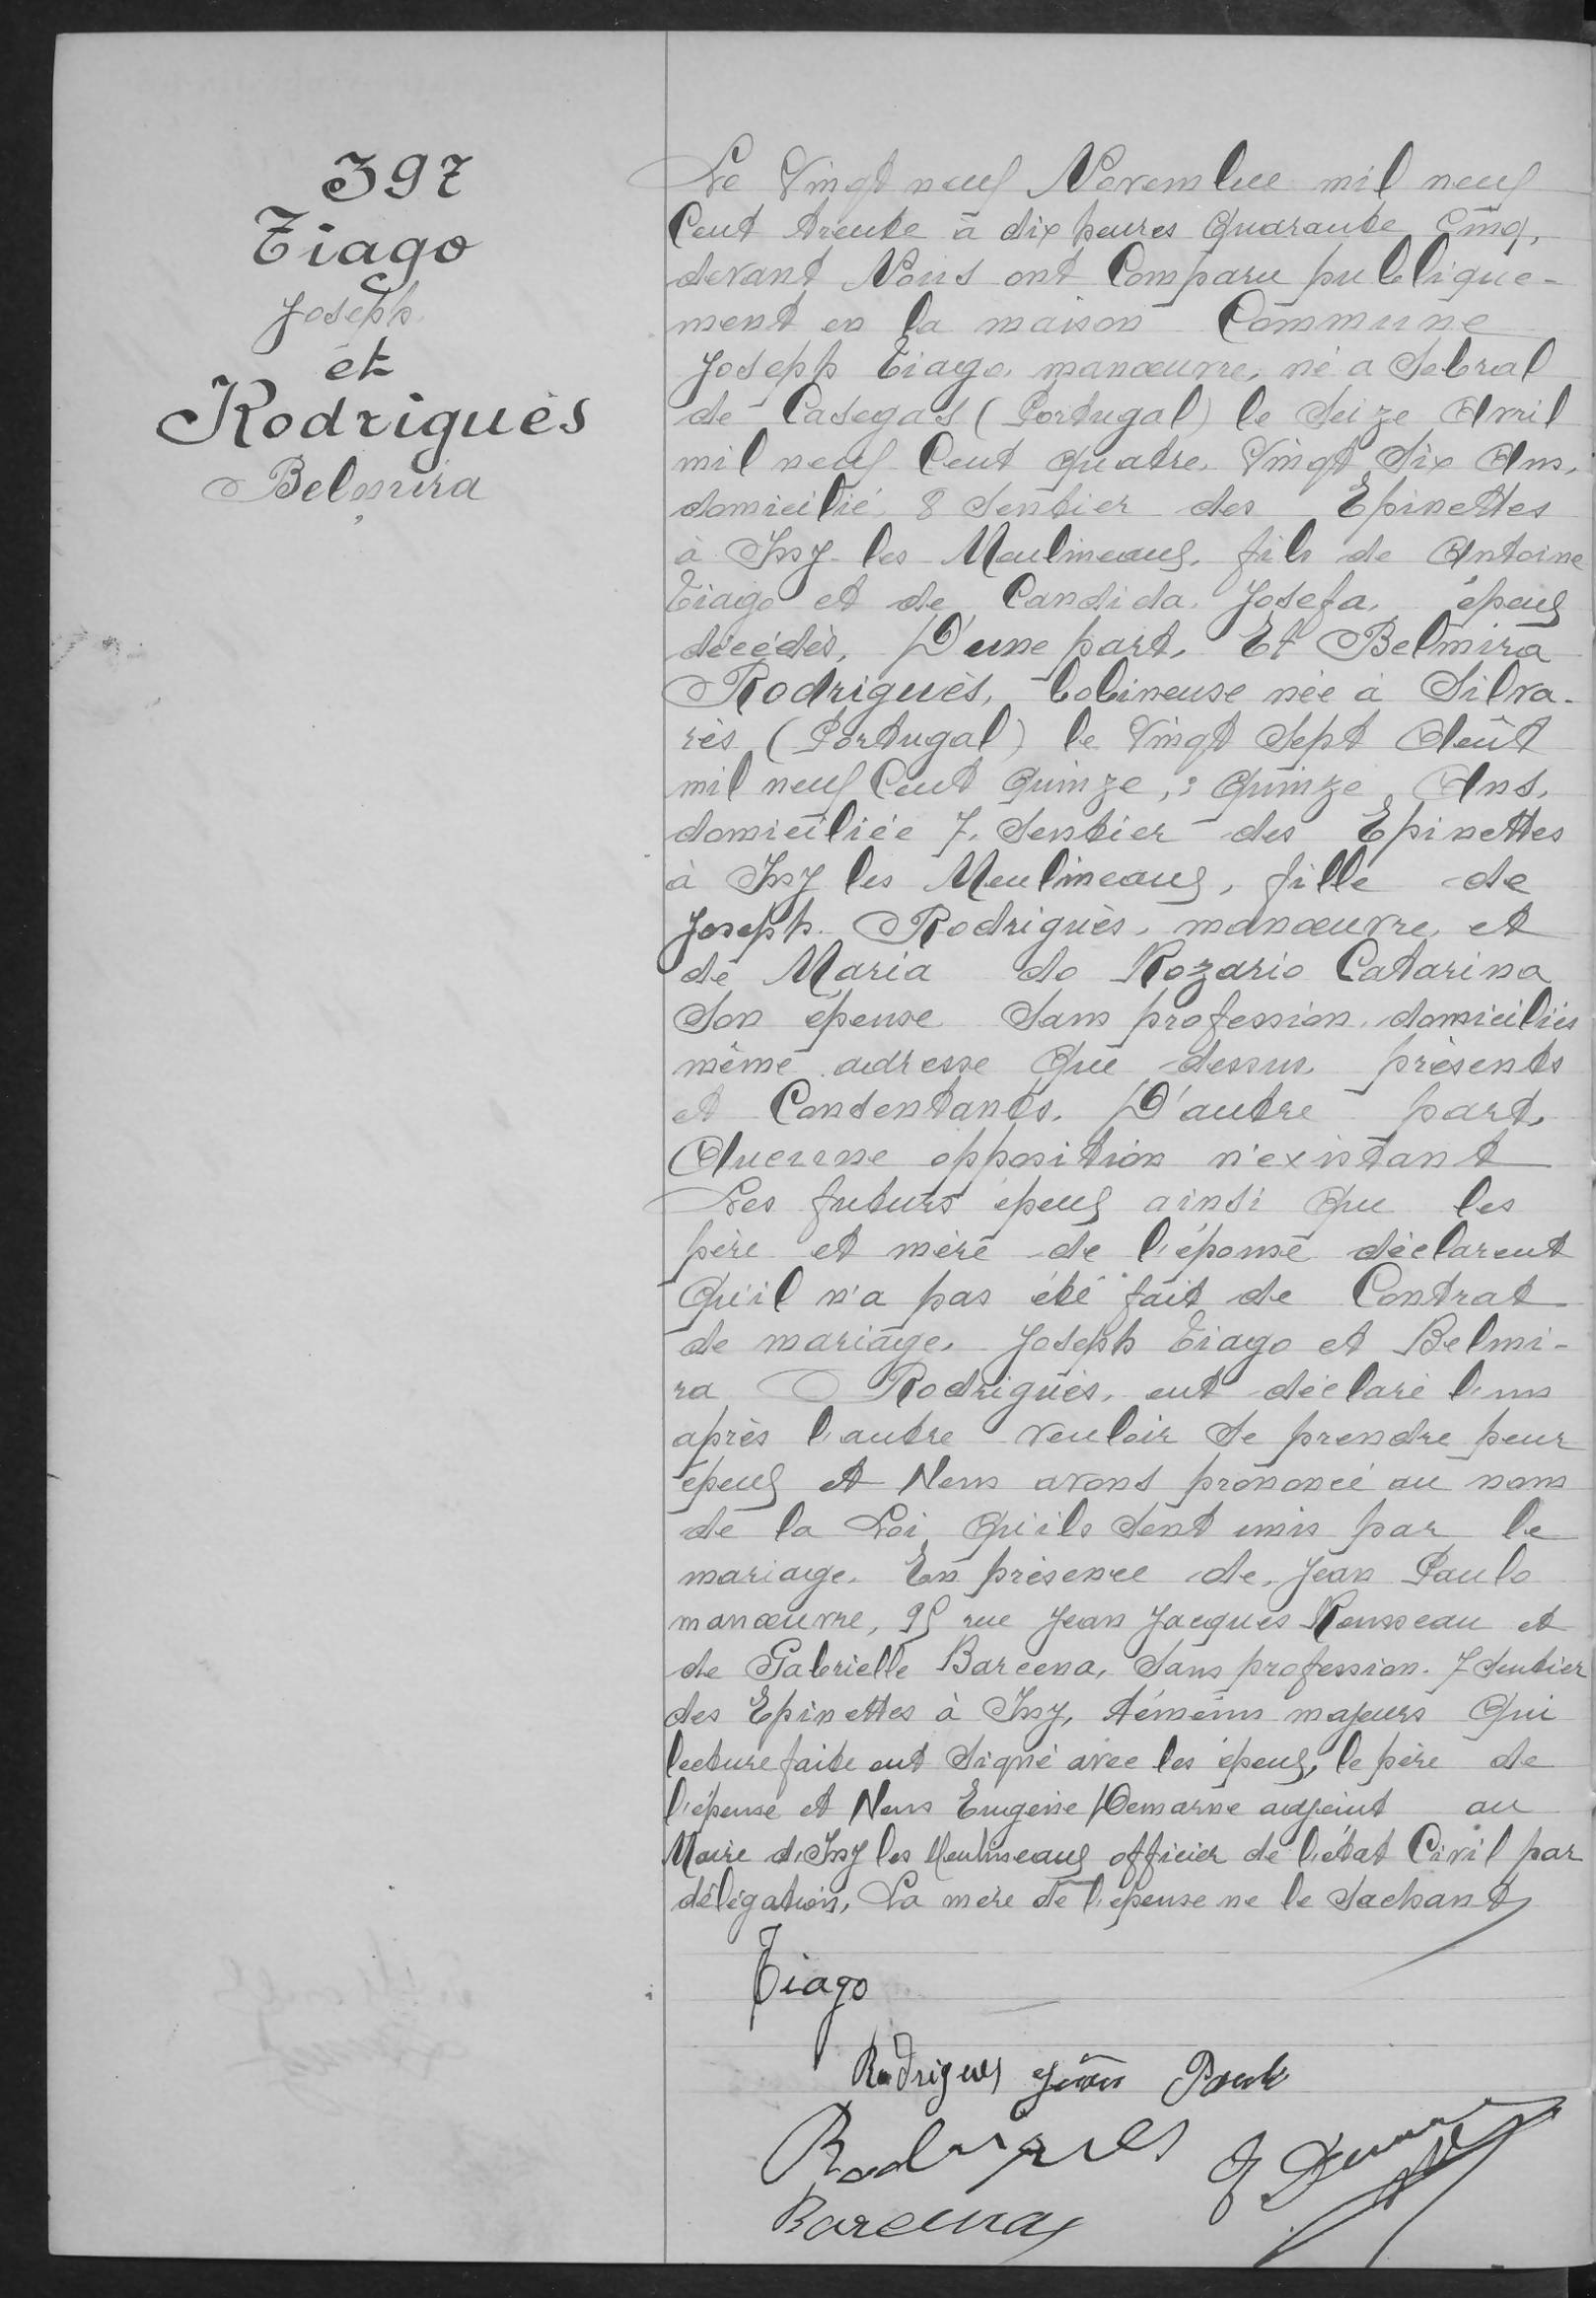397
Tiago
Joseph
et
Rodriguès
Belossira
Le Vingt neuf Novembre mil neuf
cent trente à dix heures quarante cinq,
devant Nous ont Comparu publique-
ment en la maison Commune
Joseph Tiago manoeuvre, né à Sebral
de Casegas (Portugal) le Seize Avril
mil neuf cent quatre, Vingt Six ans.
domicilié 8 Sentier des Epinettes
à Issy-les-Moulineaux, fils de Antoine
Tiago et de Candida Josefa, époux
décédés, D'une part. Et Belmira
Rodriguès bobineuse née à Silva
rès (Portugal) le Vingt Sept Août
mil neuf cent quinze, quinze Ans,
domiciliée 7, Sentier des Epinettes
à Issy les Moulineaux, fille de
Joseph Rodriguès, manoeuvre et
de Maria de Rozario Catarina
Son épouse. Sans profession, domiciliées
même adresse que dessus présents
et Consentants. D'autre part.
Aucune opposition n'existant
les futurs époux ainsi que les
père et mère de l'épouse déclarent
qu'il n'a pas été fait de Contrat
de mariage, Joseph Tiago et Belmi-
ra Rodriguès ont déclaré l'un
après l'autre vouloir se prendre pour
époux et Nous avons prononcé au nom
de la Loi qu'ils sont unis par le
mariage. En présence de Jean Paulo
manoeuvre, 95 rue Jean Jacques Rousseau et
de Gabrielle Bareena, sans profession. 7 sentier
des Epinettes à Issy, témoins majeurs, qui
lecture faite ont signé avec les époux, le père de
l'épouse et Nous Eugène Demarne adjoint au
Maire de Issy les Moulineaux, officier de l'état Civil par
délégation, la mère de l'épouse ne le sachant
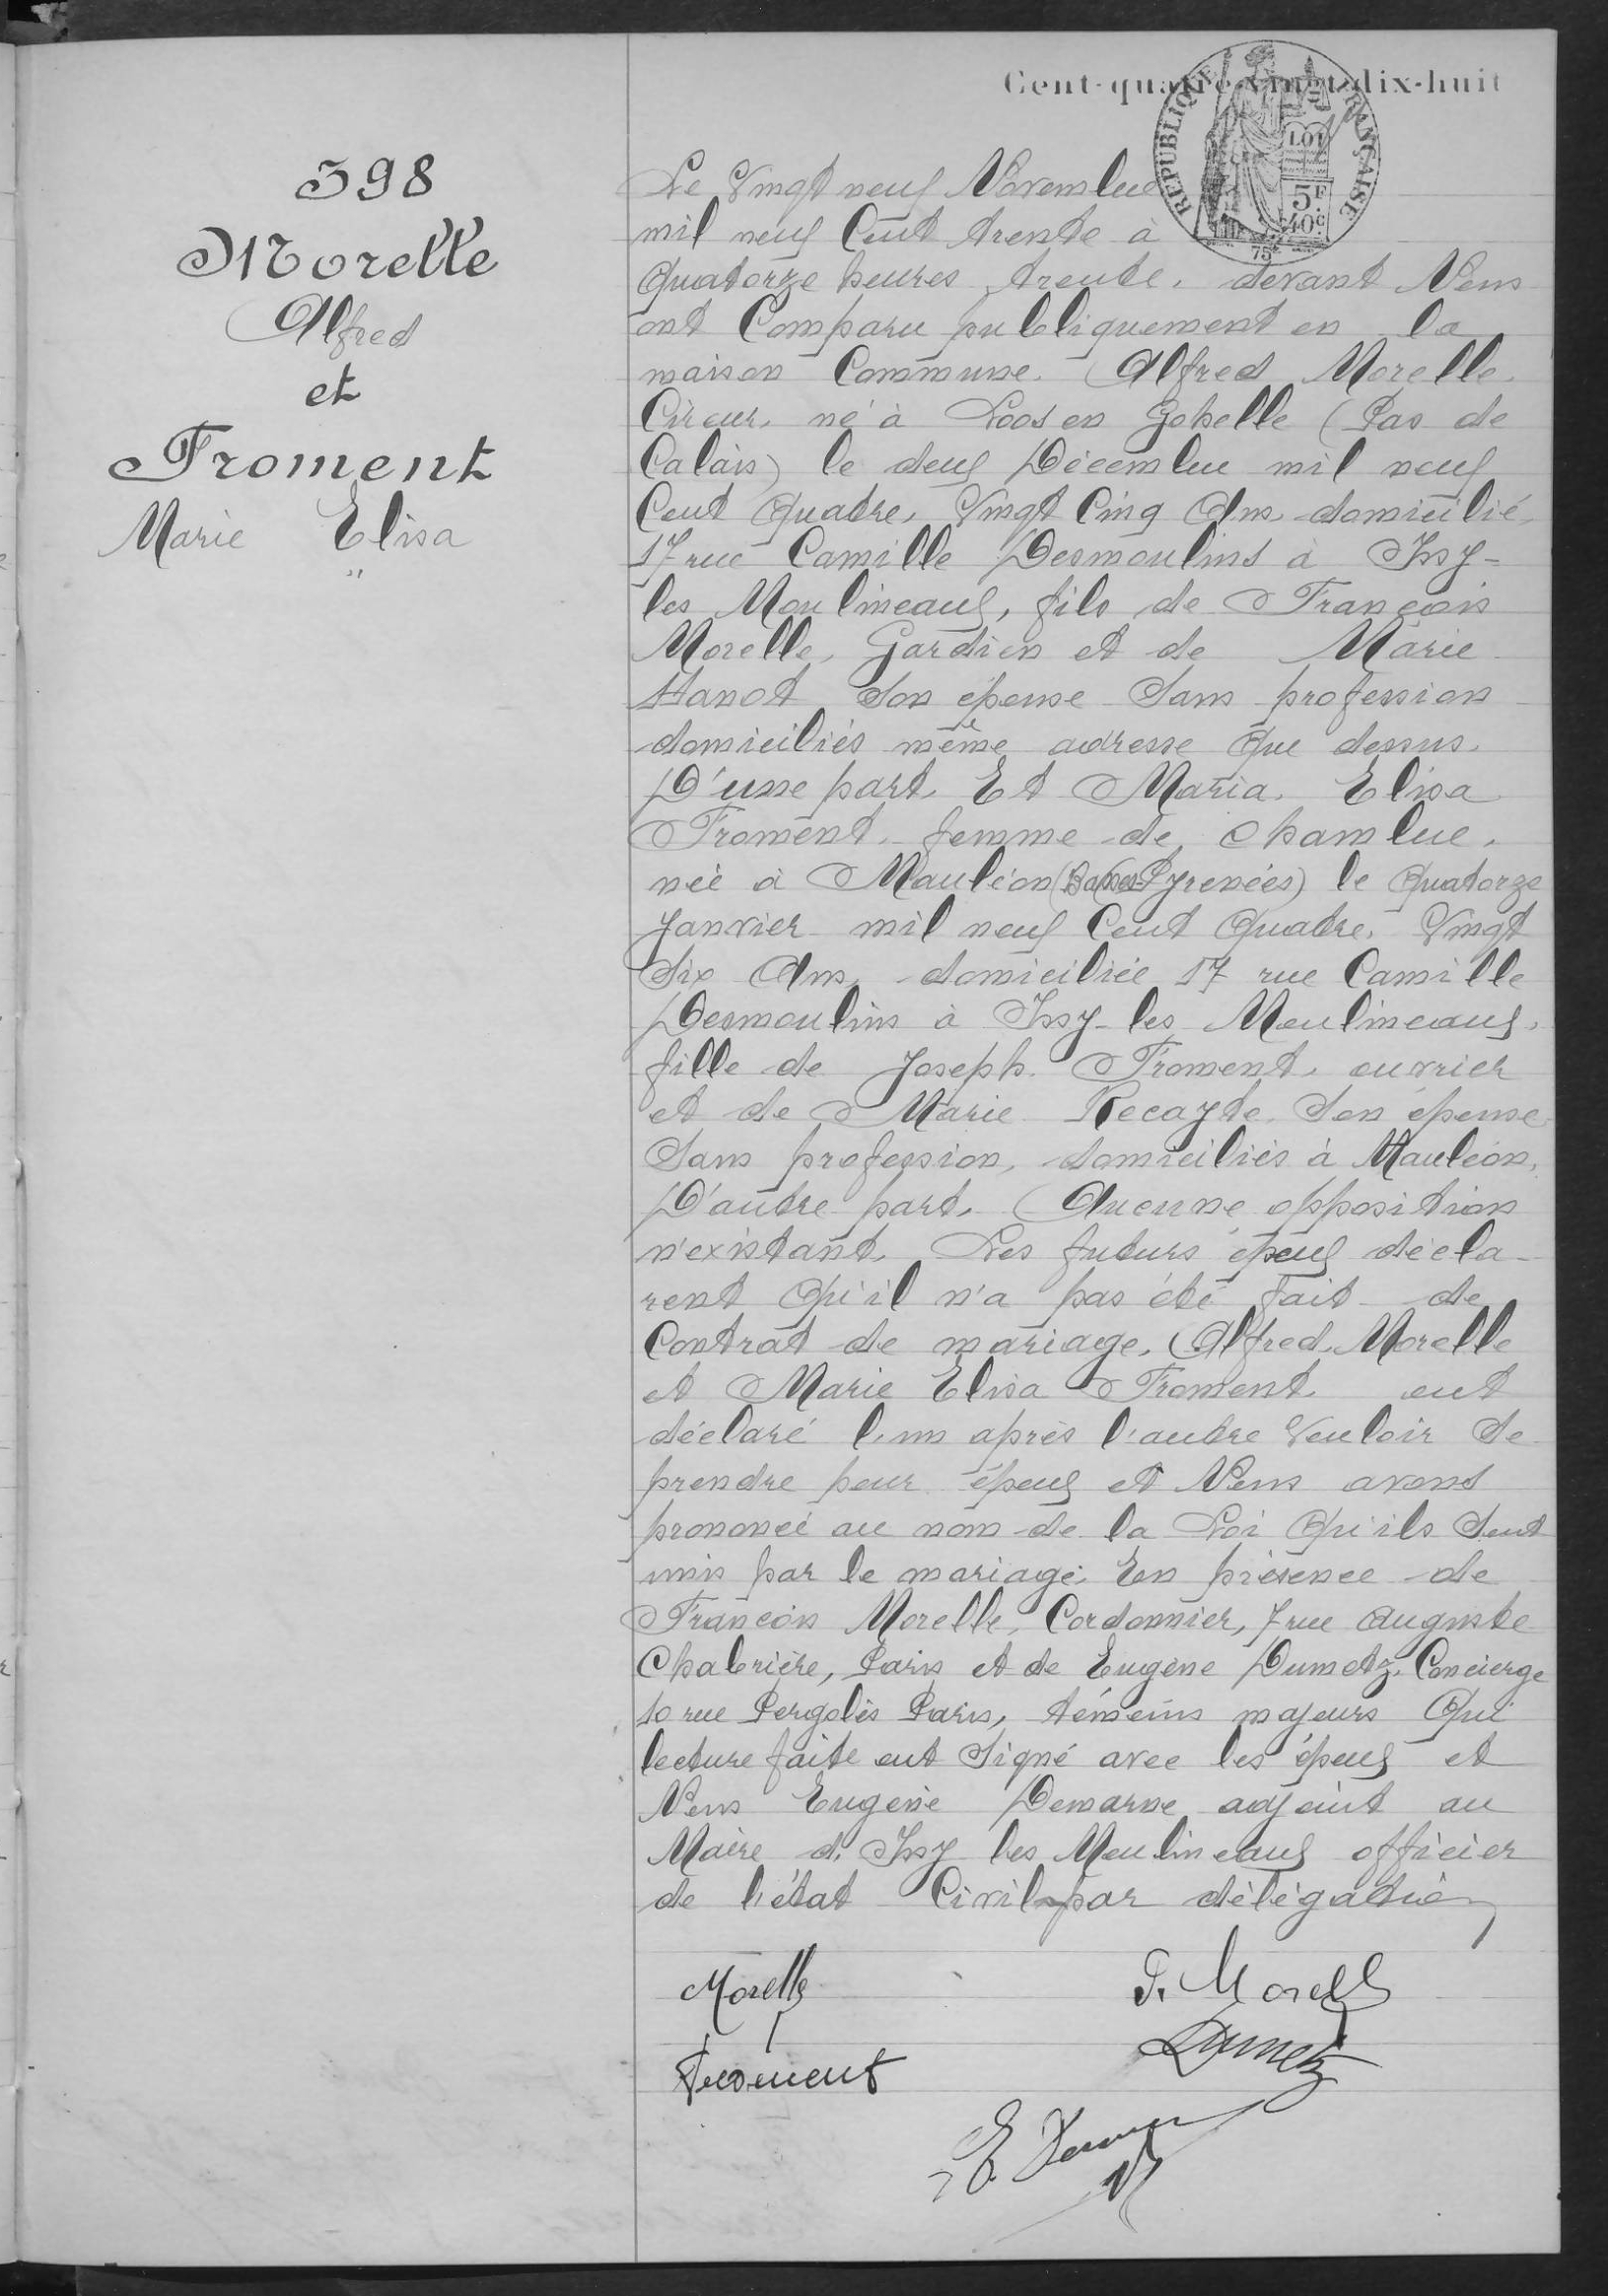398
Morelle
Alfred
et
Froment
Marie Elisa
Le Vingt neuf Novembre
mil neuf cent trente à
Quatorze heures trente, devant Nous
ont Comparu publiquement en la
maison Commune. Alfred Morelle
Cireur, né à Loos en Gobelle (Pas de
Calais) le neuf Décembre mil neuf
Cent Quatre, Vingt Cinq Ans domicilié,
17 rue Camille Desmoulins à Issy-
les Moulineaus, fils de François
Morelle, Gardien et de Marie
Hanot son épouse Sans professions
domiciliés même adresse que dessus.
D'une part EA Maria, Elisa
Froment, femme de chambre,
née à Mauléon (Basses-Pyrenées) le Quatorze
Janvier mil neuf Cent Quatre. Vingt
Six Ans domiciliée 17 rue Camille
Desmoulins à Issy-les-Moulineaux
fille de Joseph Froment ouvrier
et de Marie Recayde Son épouse
sans profession, domiciliés à Mauléon
D'autre part, Aucune opposition
n'existant. Les futurs époux décla-
rent Qu'il n'a pas été fait de
Contrat de mariage. Alfred Morelle
et Marie Elisa Froment ont
déclaré l'un après l'autre vouloir se
prendre pour époux et Nous avons
prononcé au nom de la Loi Qu'ils sont
unis par le mariage; En présence de
François Morelle, Cordonnier, 7 rue Auguste
Chabirère, Paris et de Eugène Dumet; Concierge
10 rue Pergolès Paris, témoins majeurs Qui
lecture faite ont signé avec les époux et
Nous Eugène Demarsse adjoint au
Maire d'Issy les Moulineaux officier
de l'état Civil par délégation.